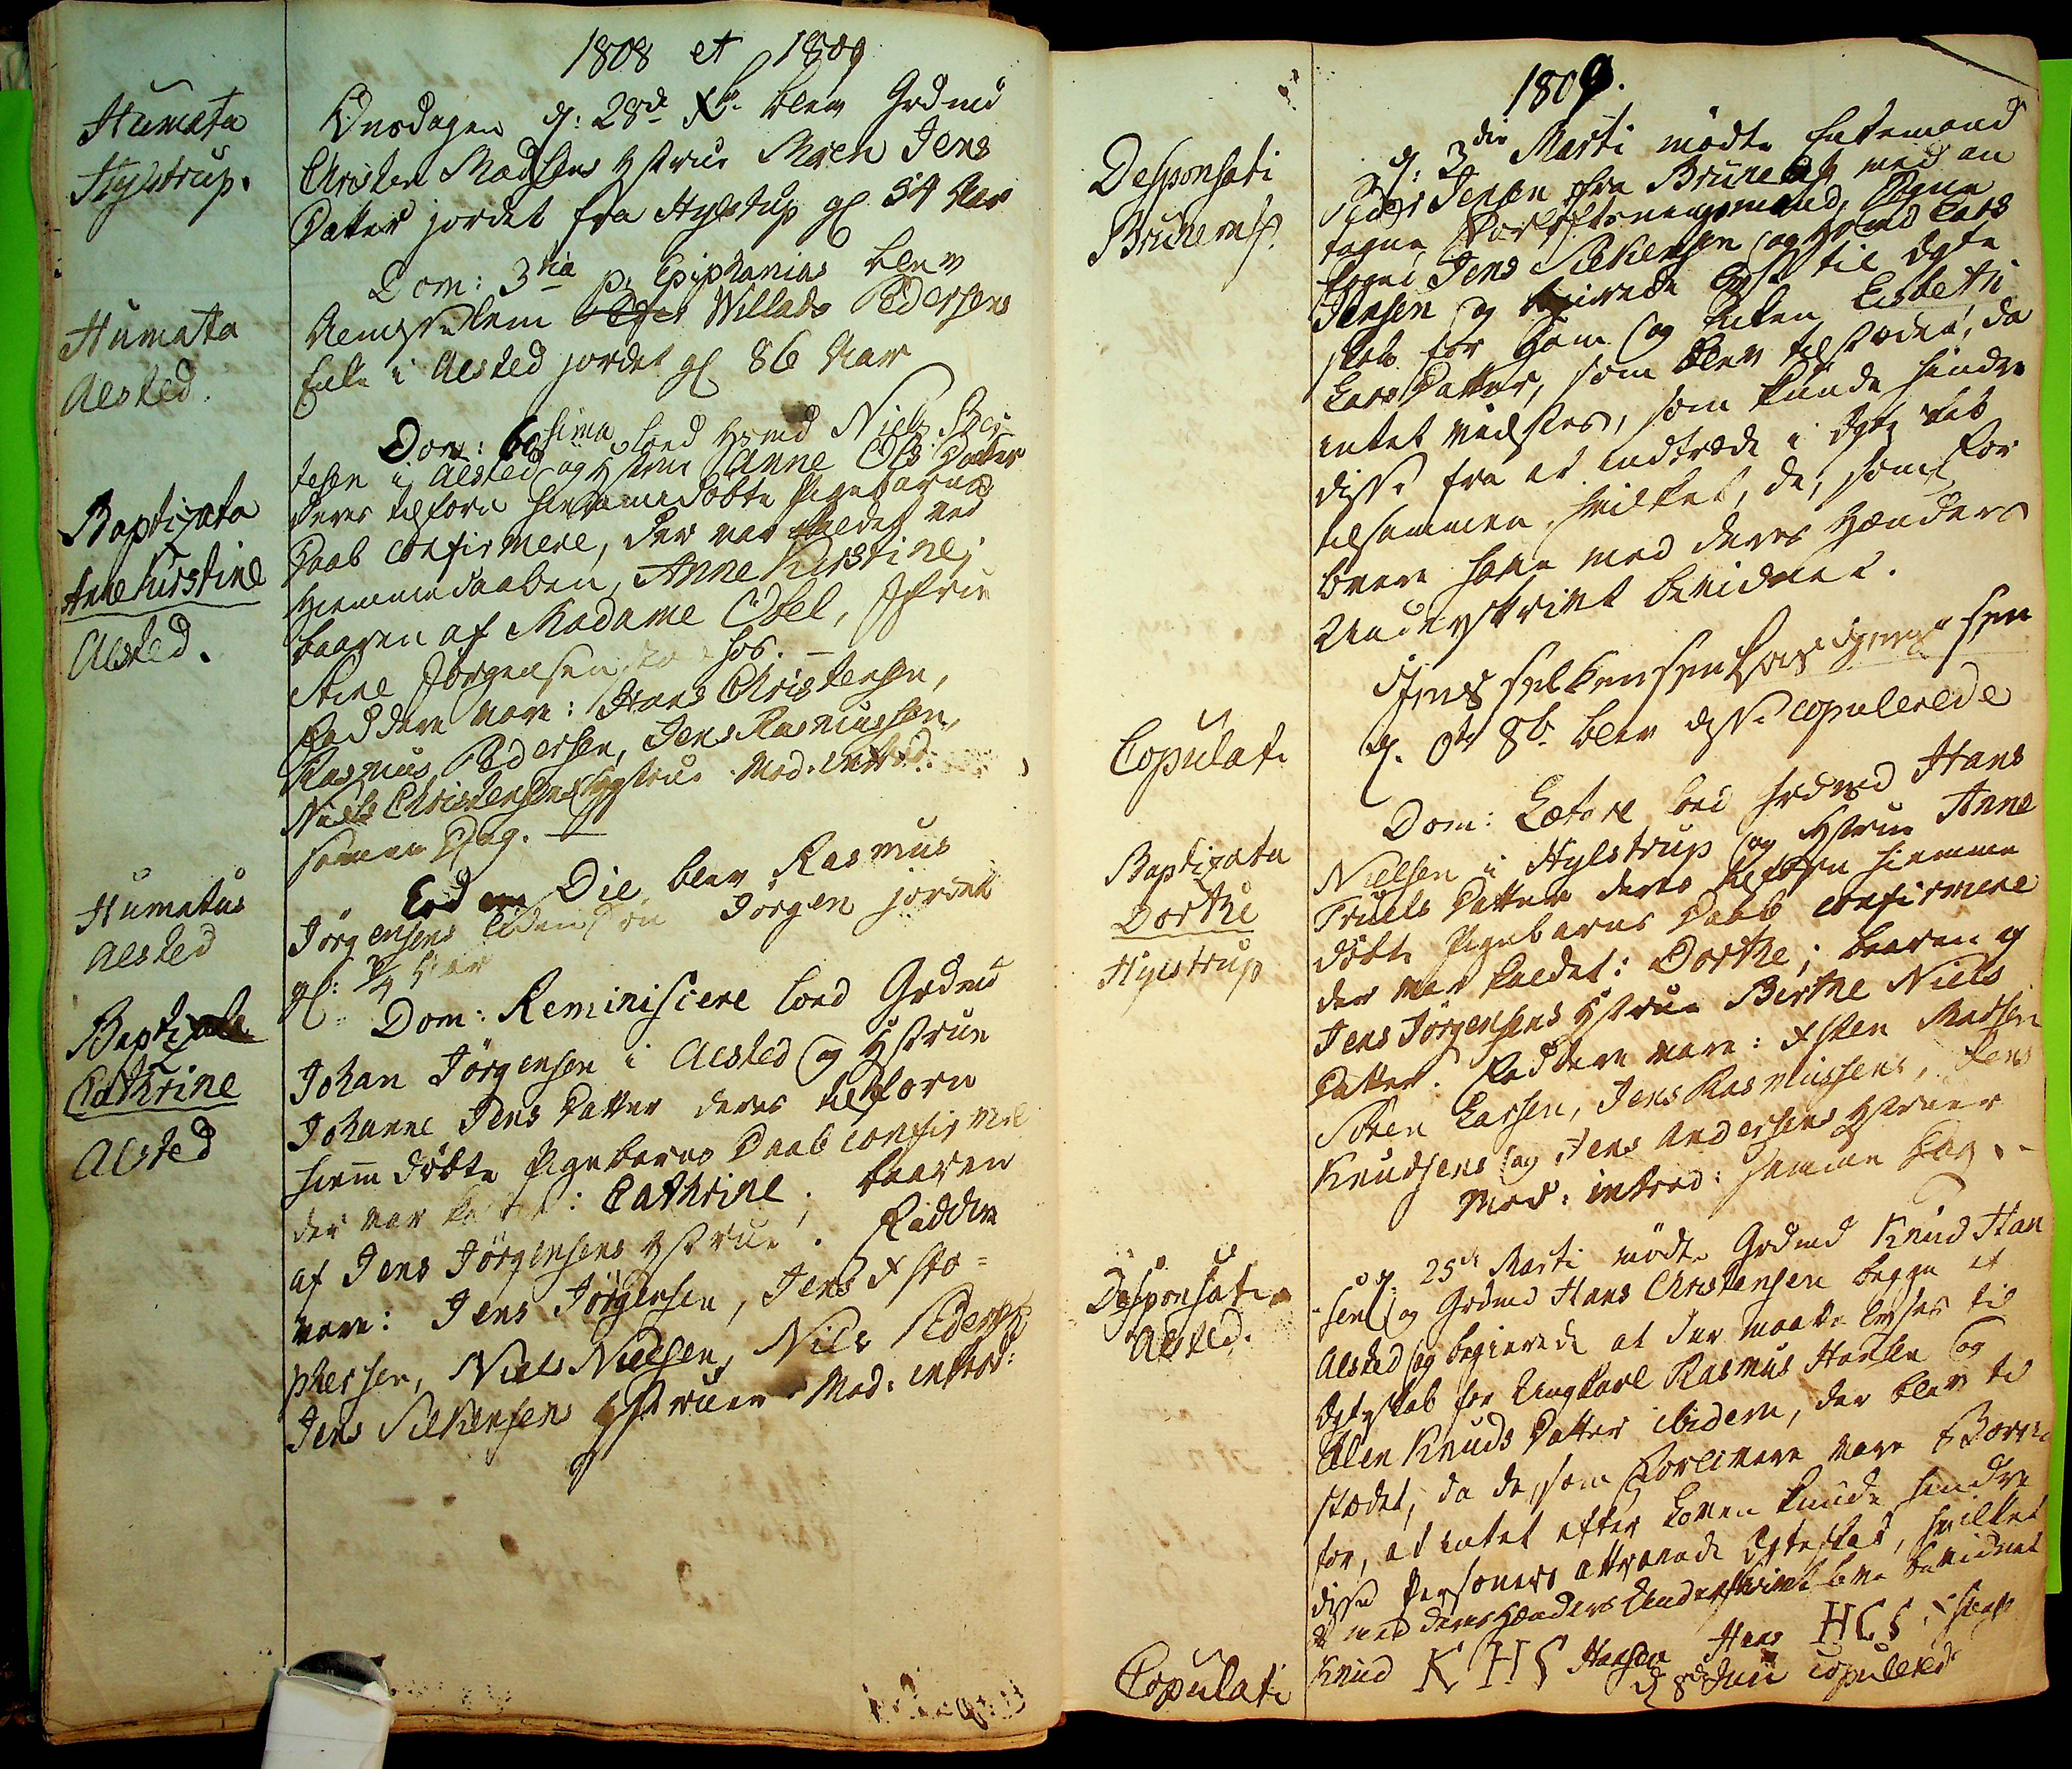Humata
Hylstrup.
Hum,ata
Alsted.
Baptizata
Anne Kirstine
Alsted.
Humatus
Alsted
Baptizata
Cathrine
Alsted
1808 et 1809
Onsdagen d: 28de Xbr blev Grdmd
Christen Madsens Hstrue Maren Jens
Datter jordet fra Hylstrup gl 54 Aar.
Dom: 3tia p. Epiphanias blev
Almisselem Peder Willads Pedersens
Enke i Alsted jordet gl 86 Aar
Dom: 60sima loed Hsmd Niels ##
##esen i Alsted, og Hustrue Anne Ols Datter
deres tilforn hiemdøbte Pigebarns
Daab confirmere, der var kaldet ved
Hiemmedaaben, Anne Kirstine
baaren af Madame Osel, Jfrue
Stine Jørgensen stoed hos.
Fadere vare: Hans Christensen,
Rasmus Pedersen, Jens Rasmussen
Niels Christensens Hstrue Mod: introd:
samme Dag.
Eodem Die blev Rasmus
Jørgensens liden Søn Jørgen jordet
gl: ¾ Aar
Dom: Reminiscere lod Grdmd
Johan Jørgensen i Asted og Hstrue
Johanne Jens Datter deres tilforn
hiemmdøbte Pigebarns Daab confirmere
der v ar kaldet: Catrine; baaren
af Jens Jørgensens Hstrue. Faddere
vare: Jens Jørgensen, Jens Xsto¬
phersen, Niels Nielssen, Niels Pedersen
Jens Silkensens Hstrue Mod: introd:
Desponsati
Brunem##
Copulatiu
Baptizata
Dorthe
Hylstrup
Desponsati
Alsted.
Copulati
1809.
d. 3die Marti mødte Enkemand
Peder Jensen fra Brunem## med an
tagne Forlovmand, Sogne
foged Jens Silkensen og Hsmd Lars
Jensen og begierede lyst til Ægte
skab for Ham og Enken Lisbeth
Lars Datter, som blev tilstedet, da
intet vidstes, som kunde hindre
disse fra at indtræde i Ægteskab
tilsammen, hvilket, de, som For
lovere have med deres Hænders
Underskrivt bevidnet
Jens Silkensen Lars Jensen
d: Ote 8b blev disse copulerede
Dom: Lætare loed Grdmd Hans
Nielsen i Hylstrup og Hstrue Anne
Truels Datter deres tilforn hiemme
døbte Pigebarns Daab confirmere
der var kaldet: Dorthe; baaren af
Jens Jørgensens Hstrue Birthe Niels
Datter. Faddere vare. Xsten Madsen
Søren Larsen, Jens Rasmussens, Jens
Knudsens og Jens Andersens Hstruer
Mod: introd: samme Dag.
d: 25de Marti mødte Grdmd Knud Han
sen og Grdmd Hans Christensen begge af
Alsted og begierede at der maatte lyses til
Ægteskab for Ungkarl Rasmus Hansen og
Ellen Knuds Dattere ibidem, det blev til
stædet, da de som Forlovere vare Borgen
for, at intet efter Loven kunde hindre
disse Personers attraade Ægteskab, hvilke
de med deres Hænders Underskrivt have bevidnet
Knud K H S Hansen Hans H C S Xstensen
d 8de Juni copulered
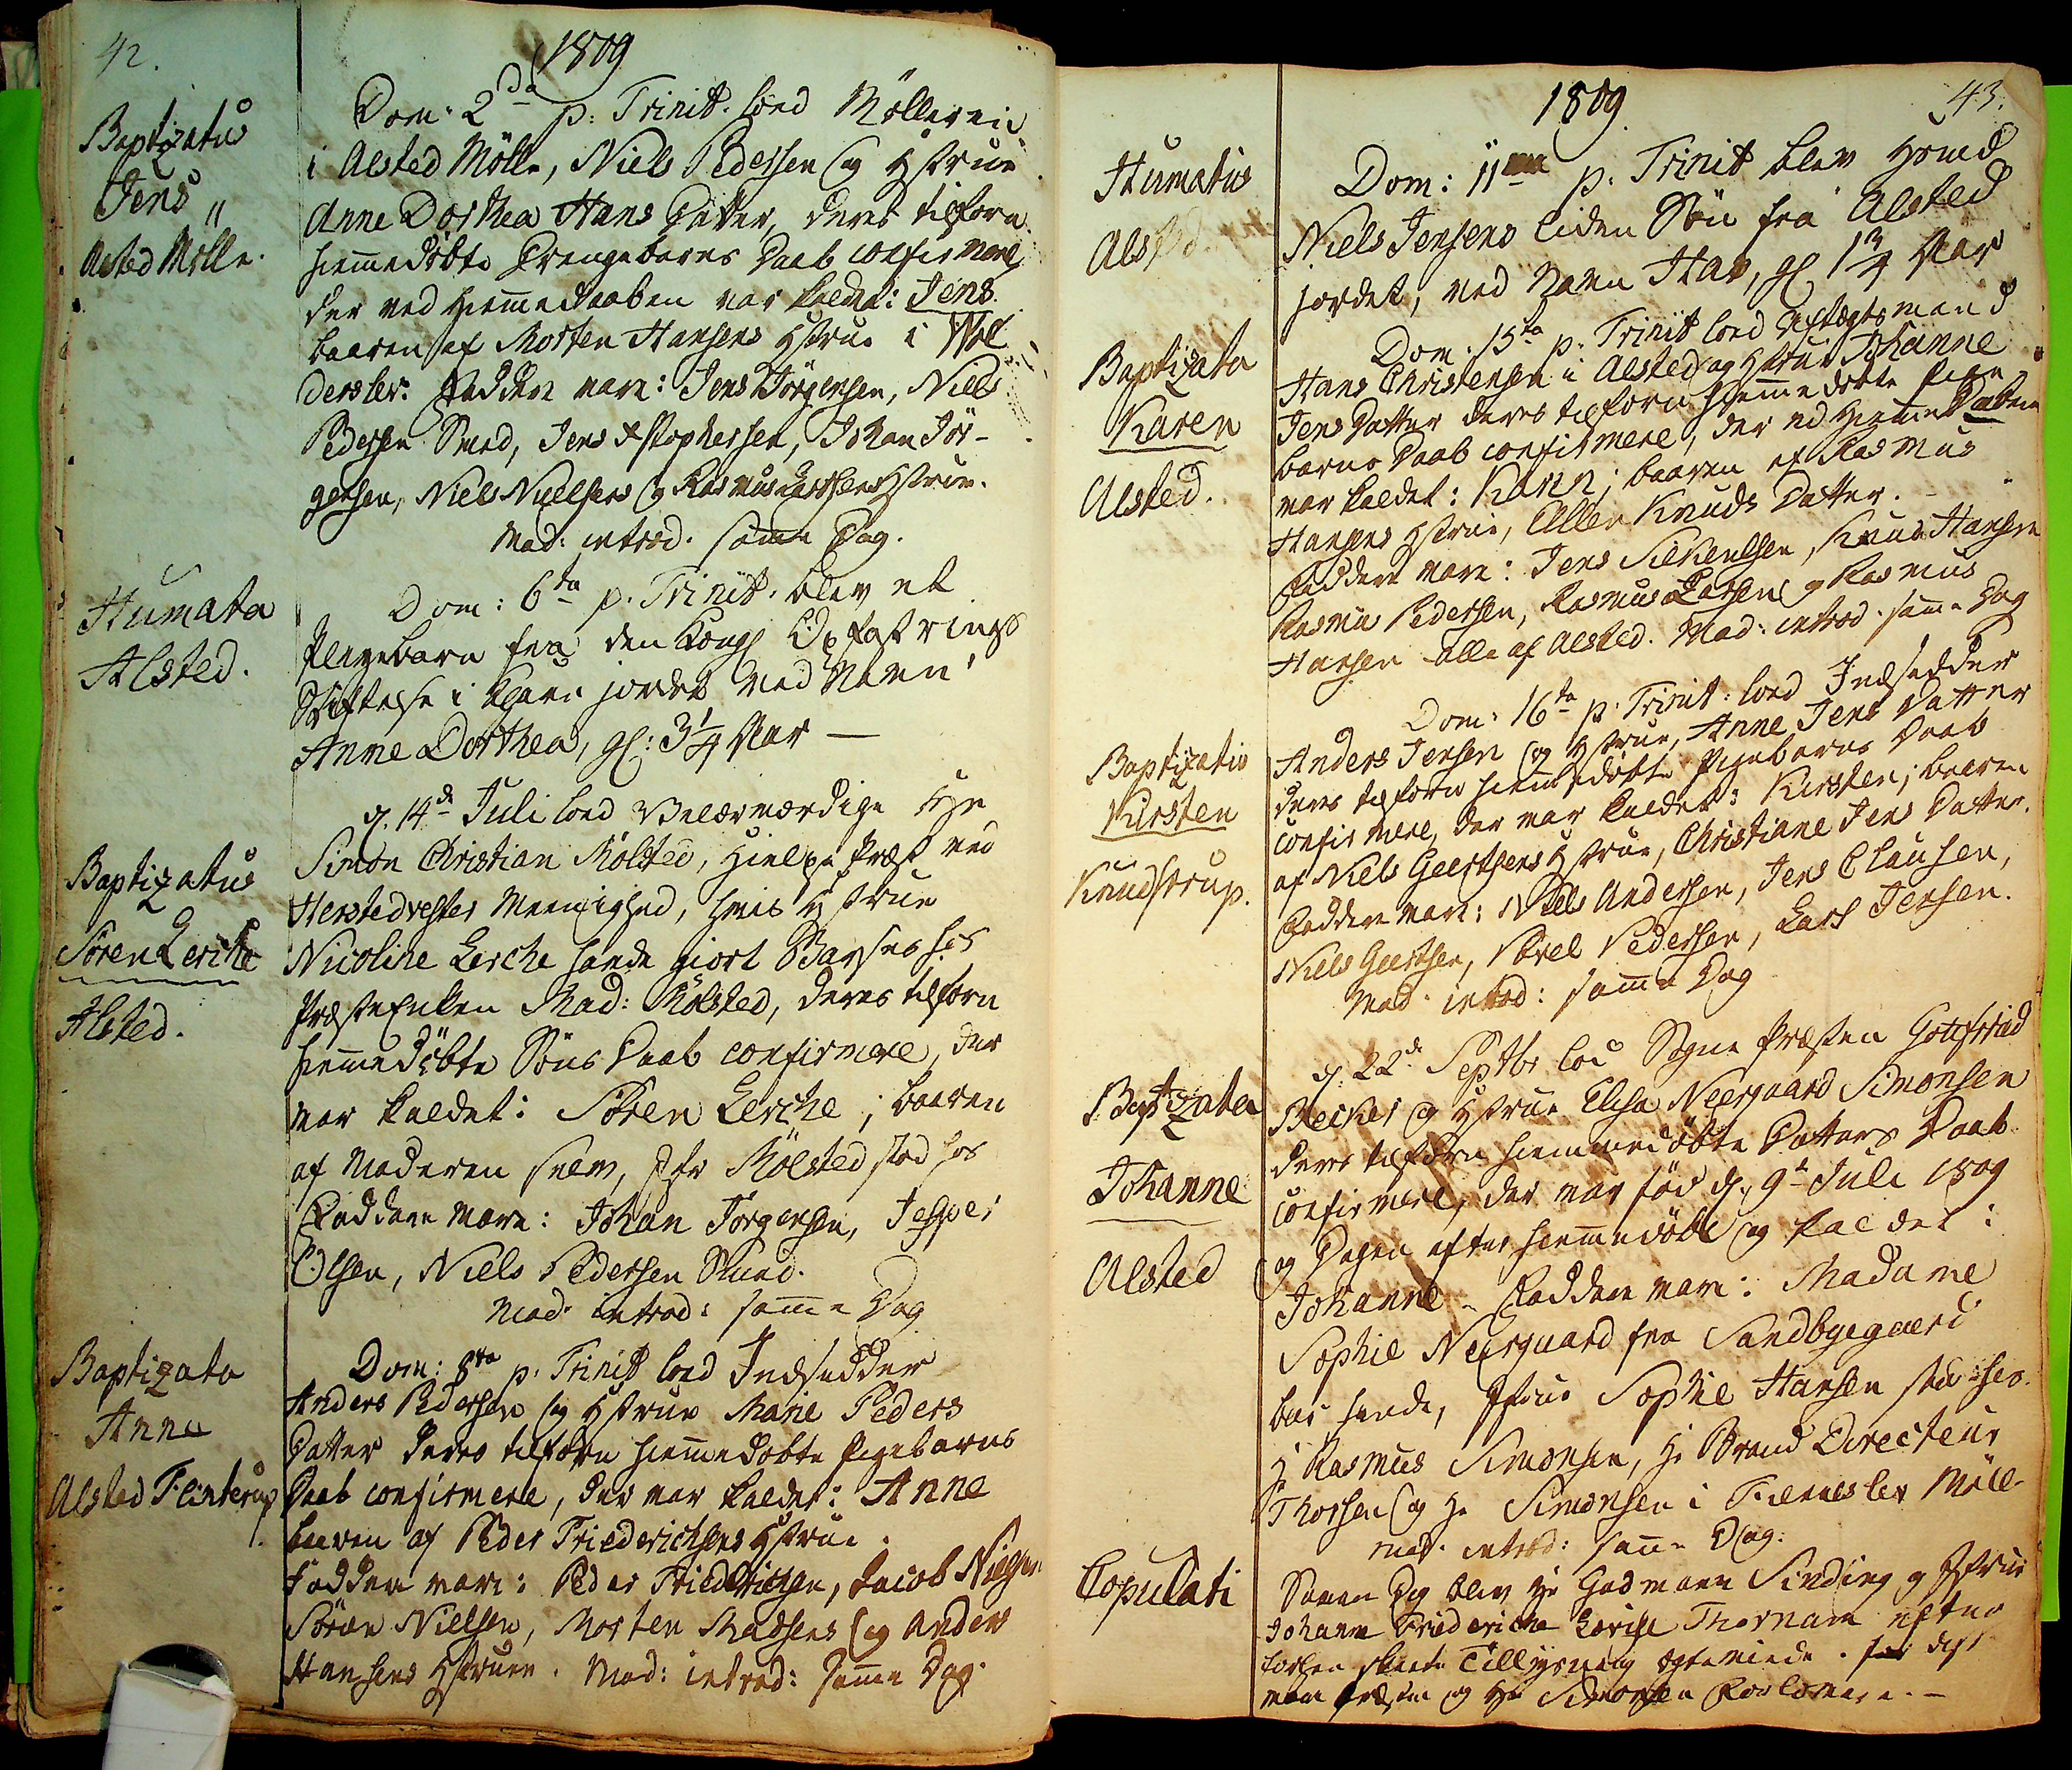42.
Baptizatus
Jens
Alsted Mølle.
Humata
Alsted.
Baptizatus
Søren Lerche
Alsted.
Baptizata
Anna
Alsled Flinterup
1809
Dom: 2da p: Trinit. loed Mølleren
i Alsted Mølle, Niels Pedersen og Hstrue
Anne Dorthe Hans Datter, deres tilforn
hiemmedøbt Drengebarns Daab conformere
der ved Hiemmedaaben var kaldet: Jens
baaren af Morten Hansens Hstrue i Wol
derslev. Faddere vare: Jens Jørgensen, Niels
Pedersen Smed, Jens Xstophersen, Johan Jør¬
gensen, Niels Nielsens og Rasmus Larrsens Hstruoer.
Mod: introd: samme Dag.
Dom: 6ta p. Trinit. blev et
Plegebarn fra den Kongl Opfostrings
Stiftelse i K## jordet ved Navn
Anne Dorthea, gl: 3¼ Aar
d. 14de Juli loed Velærværdige Hr
Simon Christian Mølsted, hielpe Præst ved
Herstedvester Meenighed, hvis Hstrue
Nicoline Lerche havde giort Barsel hos
Præste Enken Mad: Mølsted, deres tilforn
hiemmedøbte Søns Daab confirmere, der
var kaldet: Søren Lerche; baaren
af Moderen selv, Jfr Mølsted stod hos
Faddere vare: Johan Jørgensøn, Jesper
Olsen, Niels Pedersen Smed.
Mod. introd: samme Dag
Dom: 8ta p. Trinit: loed Indsidder
Anders Pedersen og Hstrue Marie Peders
Datter deresr tilforn hiemmedøbte Pigebarns
Daab confirmere, der var kaldet: Anne
baaren af Peder Friderichsens Hstrue
Faddere vare: Peder Friderichsen, Jacob Nielsen
Søren Nielsen, Morten Madsens og Anders
Hansens Hstrure. Mod: introd: samme Dag.
Hunatos.
Alsted
Baptizata
Karen
Alsted.
Baptizatus.
Kirsten
Knudstrup
Baptizata
Johanne
Alsted
Copulati
43.
1809.
Dom: 11ma p. Trinit blev Hsmd
Niels Jensens liden Søn fra Alsted
jordet ved Navn Hans, gl 1¾ Aar
Dom: 15ta p: Trinit loed Aftægtsmand
Hans Christensen i Alsted og Hstrue Johanne
Jens Datter deres tilforn hiemmedøbte Pige
barns Daab confirmere, der ved Hiemmedaaben
var kaldet: Karen, baaren af Rasmus
Hansens Hstrue, Ellen Knuds Datter
Faddere vare: Jens Silkensen Knud Hansen
Rasmus Pedersen, Rasmus Larsen og Rasmus
Hansen alle af Vested. Mod: introd. samme Dag
Dom: 16ta p: Trin: loed Indsidder
Anders Jensen og Hstrue, Anne Jens Datter
deres tilforn hiemmedøbte Pigebarns Daab
confirmare, der var kaldet: Kirsten; baaren
af Niels Geertsens Hstrue, Christiane Jens Datter.
Faddere vare: Niels Andersen, Jens Clausen,
Niels Geertsen, Povel Pedersen, Lars Jensen.
Mod: introd: samme Dag
d: 22de Septbr: lod Sogne Præsten Gottfried
Becker og Hstrue Elisa Neergaard Simonsen
deres tilforn hiemmedøbte Datters Daab
confirmerede, det var fød d: 9de Juli 1809
og Dagen efter hiemmedøbt og kaldet:
Johanne. Faddere vare. Madame
Sophie Neergaard fra Sandbyegaard
bar hende, Jfrue Sophie Hansen stod hos
Hr Rasmus Simonsen, Hr Brand Directeur
Thorsen og Hr Simonsen i Fienneslev Mølle
Mod: introd: samme Dag:
Saaen Dag blev Hr Gadmann Sinding og Hstrue
Johanne Frideriche Lerche Thoman efter
forhen sk##te Tillysning ægteviede. for disse
var Præsten og Hr Simonsen Forlovere.